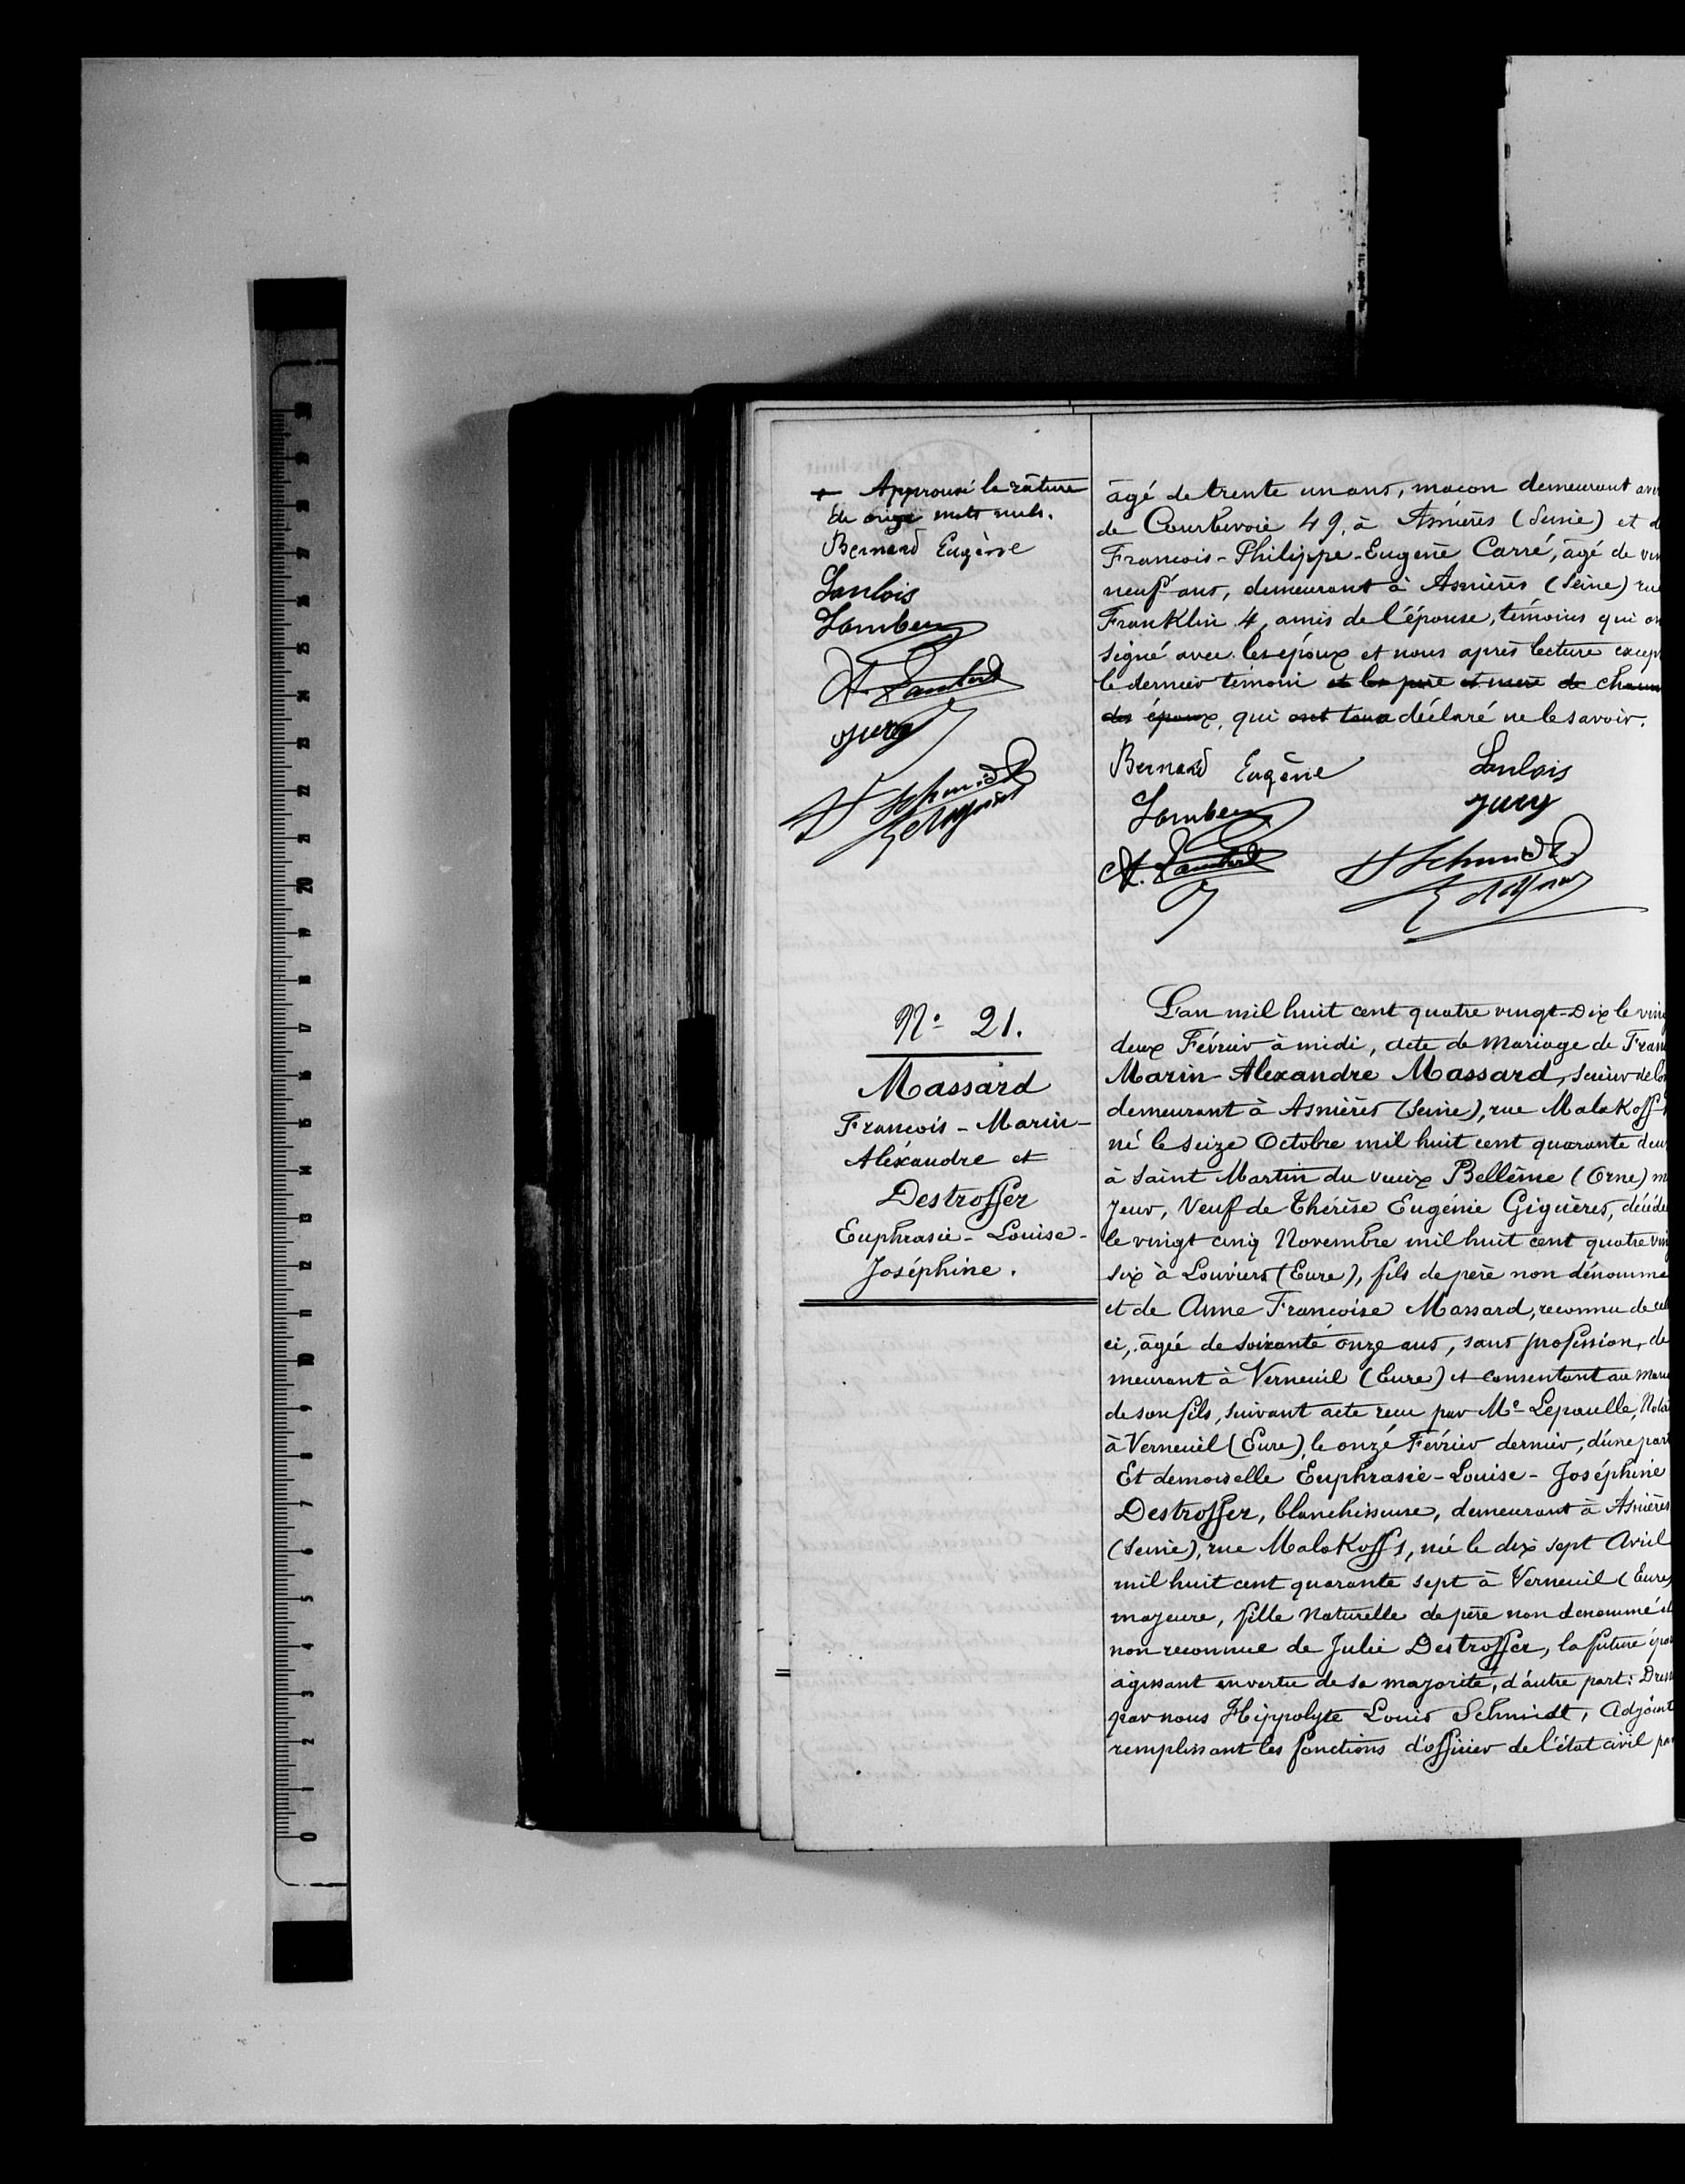âgé de trente un ans, maçon demeurant ave
de Courbevoie 49, à Asnières (Seine) et de
François-Phillipe-Eugène carré, âgé de vin
neuf-ans, demeurant à Asnières (Seine) rue
Franklin 4, amis de l'épouse, témoins qui on
signé avec lesépoux et nous après lecture except
le dernier témoin et le père et mère de chacun
des époux qui ont tous déclaré ne le savoir.
N°21
Massard
François-Marin
Alexandre et
Destroger
Euphrasie-Louise
Joséphine.
L'an mil huit cent quatre vingt-Dix le vingt
deux Février à midi, acte de Mariage de Frang
Marin-Alexandre Massard, scieur de bois
demeurant à Asnières (Seine), rue Malakoff
né le seize Octobre mil huit cent quarante deux
à Saint Martin du Vieux Bellême (Orne) m
Jeuv, Veuf de Thérèse Eugénie Giguères, décédé
le vingt cinq Novembre mil huit cent quatre ving
dix à Louviers (Eure), fils de père non dénommé
et de Anne Françoise Massard, reconnu de cel
ci, âgée de soixante onze ans, sans profession, de
meurant à Verneuil (Eure) et consentant au maria
de sonfils, suivant acte recu par M° Lepaulle, Notai
à Verneuil (Eure) le onze Février dernier, d'unepart
Et demoiselle Euphrasie-Louise-Joséphine
Destrofer, blanchisseur, demeurant à Asnières
(Seine), rue Malakoff, née le dix sept Avril
mil huit cent quarante sept à Verneuil (Eure)
majeure, fille naturelle de père non denommé et
non-reconnue de Julier Destrofer, la futur épou
agissant envertu de sa majorité, d'autre part: Dress
par nous Hyppolyte Louis Schmindt, Adjoit
remplissant les fonctions d'officier de l'état civil par
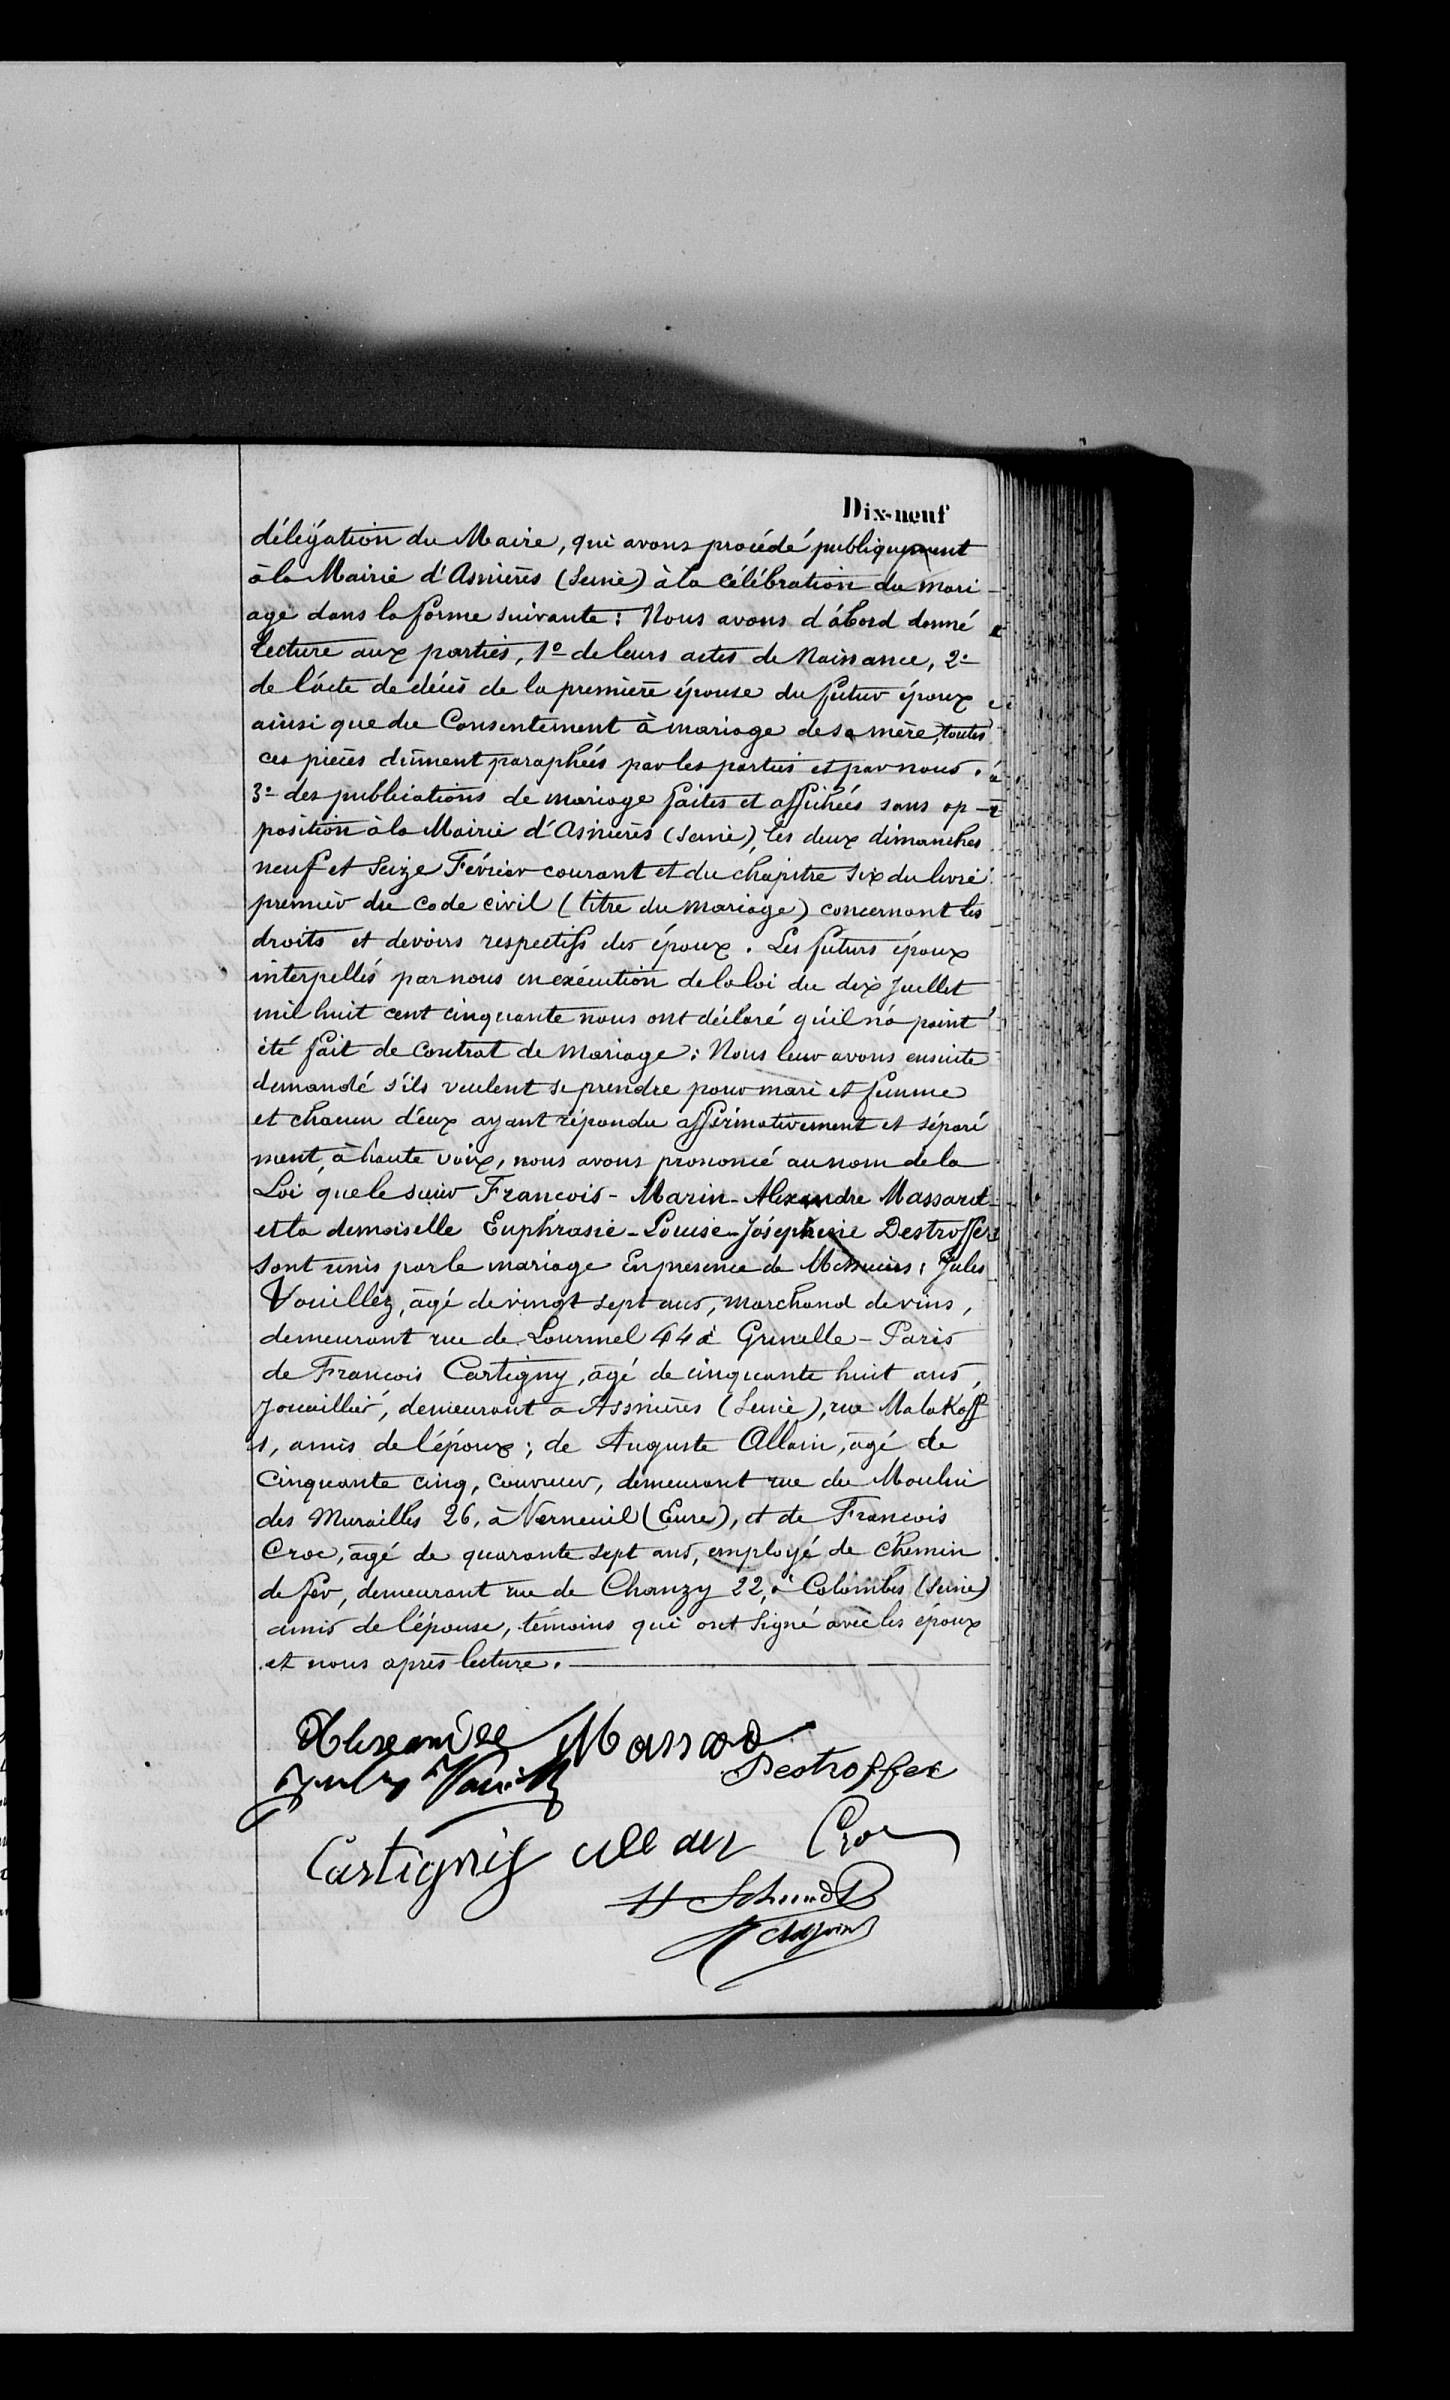délégation du Maire, qui avons procédé publiquement
à la Mairie d'Asnières (Seine) à la célébration du mari
age dans la forme suivant: Nous avons d'abord donné
lecture aux parties, 1° de leurs actes de naissance, 2°
de l'acte de décès de la première épouse du futur époux
ainsi que du Consentement à mariage des mère, toutes
ces pièces dûment paraphées par les parties et par nous,
3° des publications de mariage faites et affichées sans op-
position à la Mairie d'Asnières (Seine), les deux dimanches
neuf et seize Février courant et du chapitre six du livre
premier du Code Civil (titre du Mariage) concernant les
droits et devoir respectifs des époux. Les futurs époux
interpellés par nous en exécution de la loi du dix Juillet
mil huit cent quarante nous ont déclaré qu'il n'a point
été fait de Contrat de Mariage; Nous leur avons ensuite
démandé s'ils veulent se prendre pour mari et femme
et chacun d'eux ayant répondu affirmativement et séparé
ment à haute voix, nous avons prononcé au nom de la
Loi que le sieur François-Marin-Alexandre Massart
et la demoiselle Euphrasie-Louise-Joséphine Destroffer
sont unis par le mariage. En présence de Uchussiés: Jules
Vouillez, âgé de vingt sept ans, marchand de vins,
demeurant rue de Lourmel 44 à Grenelle-Paris
de François Cartigny, âgé de cinquante huit ans
Jouaillier, demeurant à Asnières (Seine) rue Malakoff
1, amis de l'époux; de Auguste Allain, âgé de
Cinquante cinq; couvreur, demeurant rue du Moulin
des Murailles 26, à Verneuil (Eure), et de François
Croc, âgé de quarante sept ans, employé de chemin
de fer, demeurant rue de Chanzy 22, à Colombes (Seine)
amis de l'épouse, témoins qui ont signé avec les époux
et nous après lecture. -